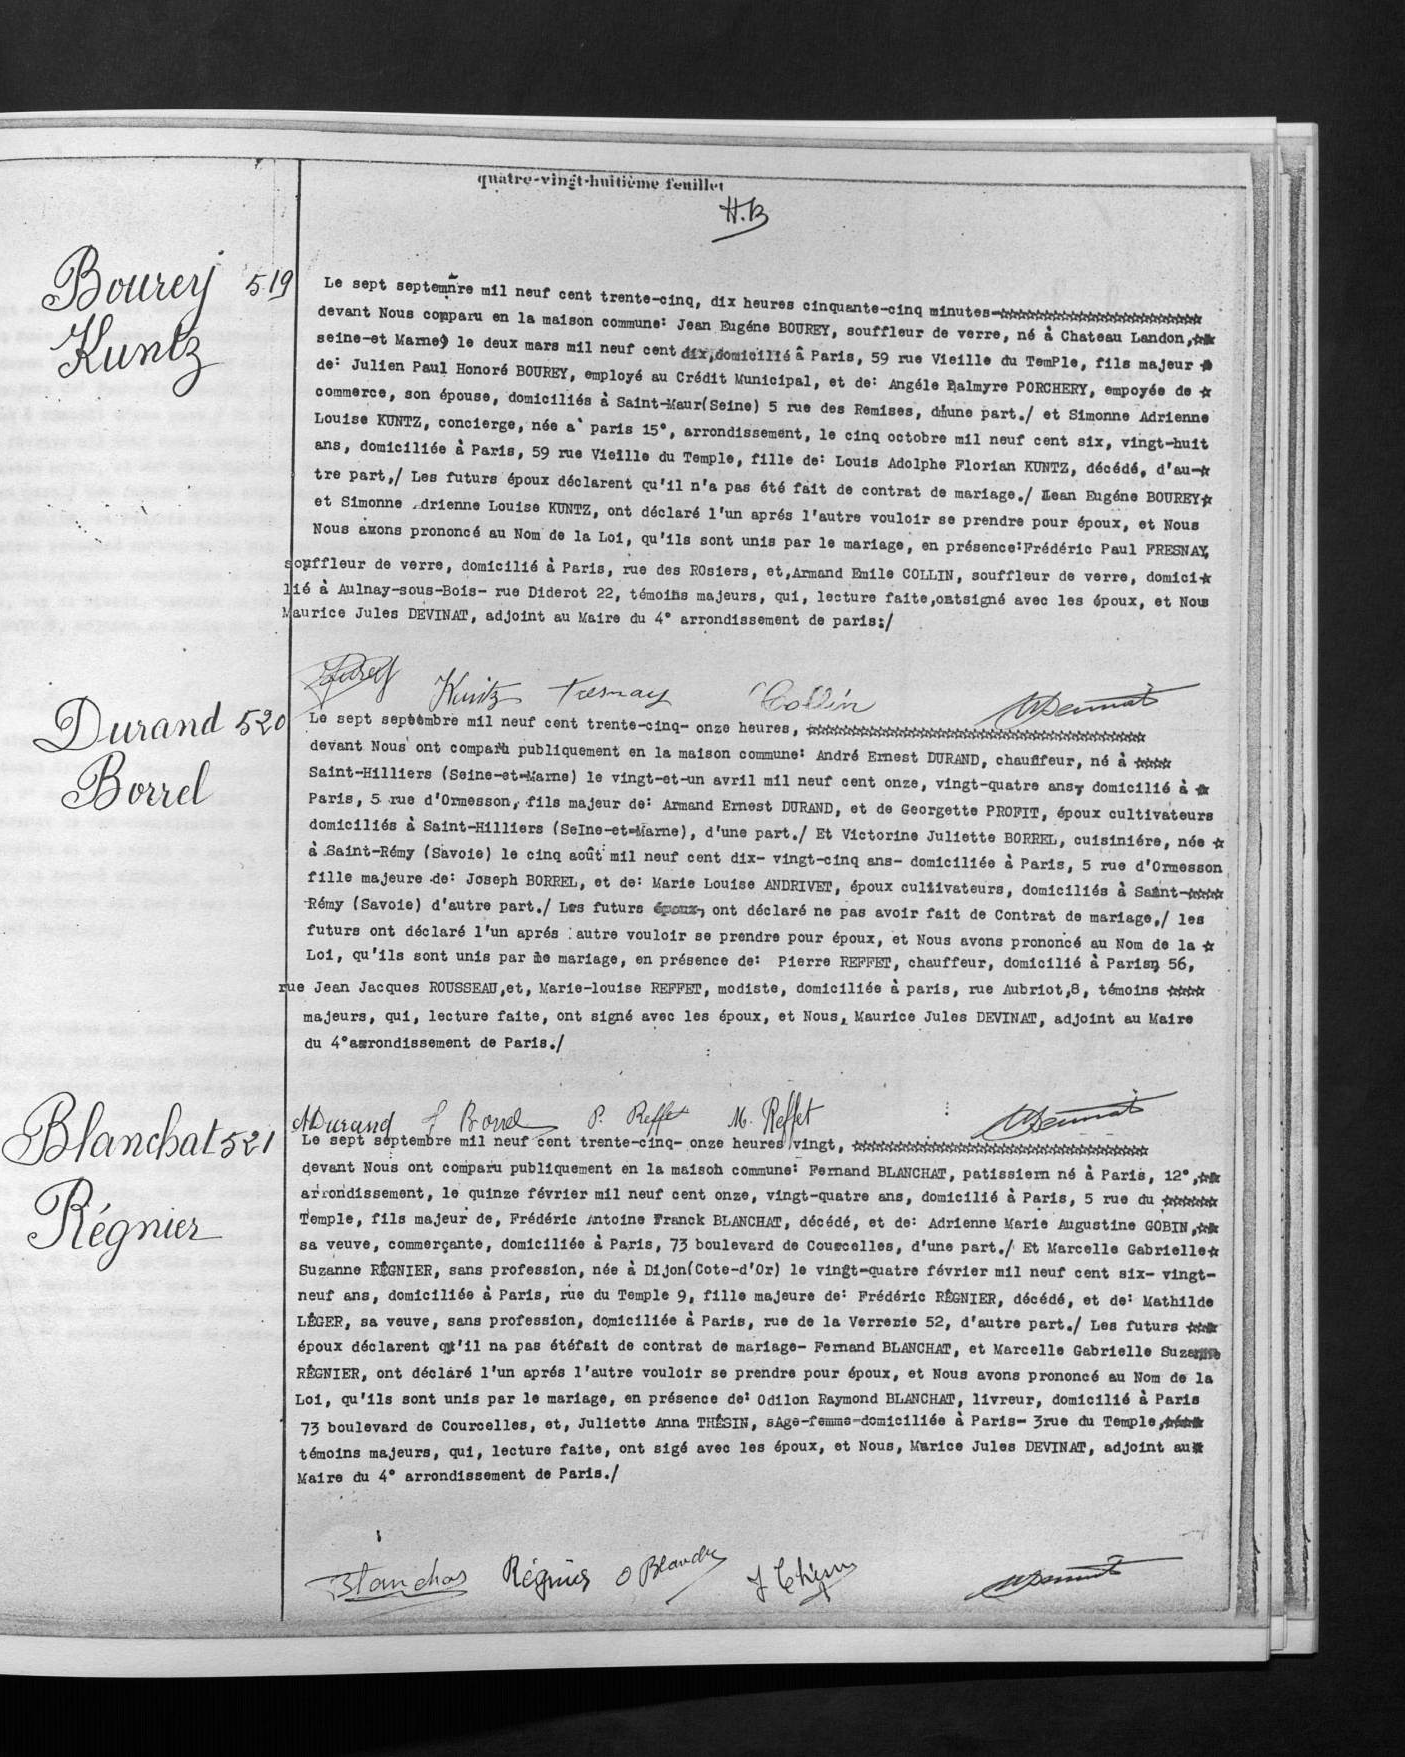Le sept septembre mil neuf cent trente-cinq, dix heures cinquante-cinq minutes -****
devant Nous comparu en la maison commune: Jean Eugène BOUREY, souffleur de verre, né à Chateau Landon, **
seine-et Marne) le deux mars mil neuf cent dix, domicilié à Paris, 59 rue Vieille du TemPle, fils majeurs *
de: Julien Paul Honoré BOUREY, employé au Crédit Municipal, et de: Angéle Palmyre PORCHERY, employée de *
commerce, son épouse, domiciliés à Saint-Maur (Seine) 5 rue des Remises, d'une part ./ et Simonne Adrienne
Louise KUNTZ, concierge, née à paris 15°, arrondissement, le cinq octobre mil neuf cent six, vingt-huit
ans, domiciliée à Paris, 59 rue Vieille du Temple, fille de: Louis Adolphe Florian KUNTZ, décédé, d'au -*
tre part ,/ Les futrs époux déclarent qu'il n'a pas été fait de contrat de mariage ./ Lean Eugéne BOUREY*
et Simonne Adrienne Louise KUNTZ, ont déclaré l'un aprés l'autre vouloir se prendre pour époux, et Nous
Nous avons prononcé au Nom de la Loi, qu'ils sont unis par le mariage, en présence: Frédéric Paul FRESNAY
souffleur de verre, domicilié à Paris, rue des R0siers, et, Armand Emile COLLIN, souffleur de verre, domici*
lié à Aulnay-sous-Bois-rue Diderot 22, témoins majeurs, qui, lecture faite, ont signé avec les époux, et Nous
Maurice Jules DEVINAT, adjoint au Maire du 4° arrondissement de paris :/
Le sept septembre mil neuf cent trente-cinq-onze heures, ****
devant Nous ont comparu publiquement en la maison commune: André Ernest DURAND, chauffeur, né à ****
Saint-Hilliers (Seine-et-Marne) le vingt-et-un avril mil neuf cent onze, vingt-quatre ans-domicilié à *
Paris, 5 rue d'Ormesson, fils majeur de: Armand Ernest DURAND, et de Georgette PROFIT, époux cultivateurs
domiciliés à Saint-Hilliers (SeIne-et-Marne), d'une part ./ Et Victorine Juliette BORREL, cuisiniére, née*
à Saint-Rémy (Savoie) le cinq août mil neuf cent dix-vingt-cinq ans-domiciliée à Paris, 5 rue d'Ormesson
fille majeure de: Joseph BORREL, et de: Marie Louis ANDRIVET, époux cultivateurs, domiciliés à Saint -****
Rémy (Savoie) d'autre part ./ Les futurs époux, ont déclaré ne pas avoir fait de Contrat de mariage ,/ les
futurs ont déclaré l'un aprés autre vouloir se prendre pour épux, et Nous avons prononcé au Nom de la *
Loi, qu'ils sont unis par me mariage, en présence de: Pierre REFFET, chauffeur, domicilié à Parisn, 56,
rue Jean Jacques ROUSSEAU, et, Marie-louise REFFET, modiste, domiciliée à paris, rue Aubriot, 8, témoins ****
majeurs, qui, lecture faite, ont signé avec les époux, et NOus, Maurice Jules DEVINAT, adjoint au Maire
du 4° arrondissement de Paris ./
Le sept septembre mil neuf cent trente-cinq-onze heures vingt; ****
devant Nous ont comparu publiquement en la maison commune: Fernand BLANCHAT, patissiern né à Paris, 12°, **
arrondissement, le quinze février mil neuf cent onze, vingt-quatre ans, domicilié à Paris, 5 rue du ****
Temple, fils majeur de, Frédéric Antoine Franck BLANCHAT, décédé, et de: Adrienne Marie Augustine GOBIN, **
sa veuve, commerçante, domiciliée à Paris, 73 boulevard de Courcelles, d'une part ./ ET Marcelle Gabrielle *
Suzanne REGNIER, sans profession, née à Dijon (Cote-d'Or) le vingt-quatre février mil neuf cent six-vingt-
neuf ans, domicilié à Paris, rue du Temple 9, fille majeure de: Frédéric REGNIER, décédé, et de: Mathilde
LEGER, sa veuve, sans profession, domiciliée à Paris, rue de la Verrerie 52, d'autre part ./ Les futurs ****
époux déclarent qu'il na pas été fait de contrat de mariage-Fernand BLANCHAT, et Marcelle Gabrielle Suzanne
REGNIER, ont déclaré l'un aprés l'autre vouloir se prendre pour époux, et Nous avons prononcé au Nom de la
Loi, qu'ils sont unis par le mariage, en présence de: Odilon Raymond BLANCHAT, livreur, domicilié à Paris
73 boulevard de Courcelles, et, Juliette Anna THESIN, sAge-femme domiciliée à Paris-3 rue du Temple, ****
témoins majeurs, qui, lecture faite, on sigé avec les époux, et Nous, Marice Jules DEVINAT, adjoint au *
Maire du 4° arrondissement de Paris ./